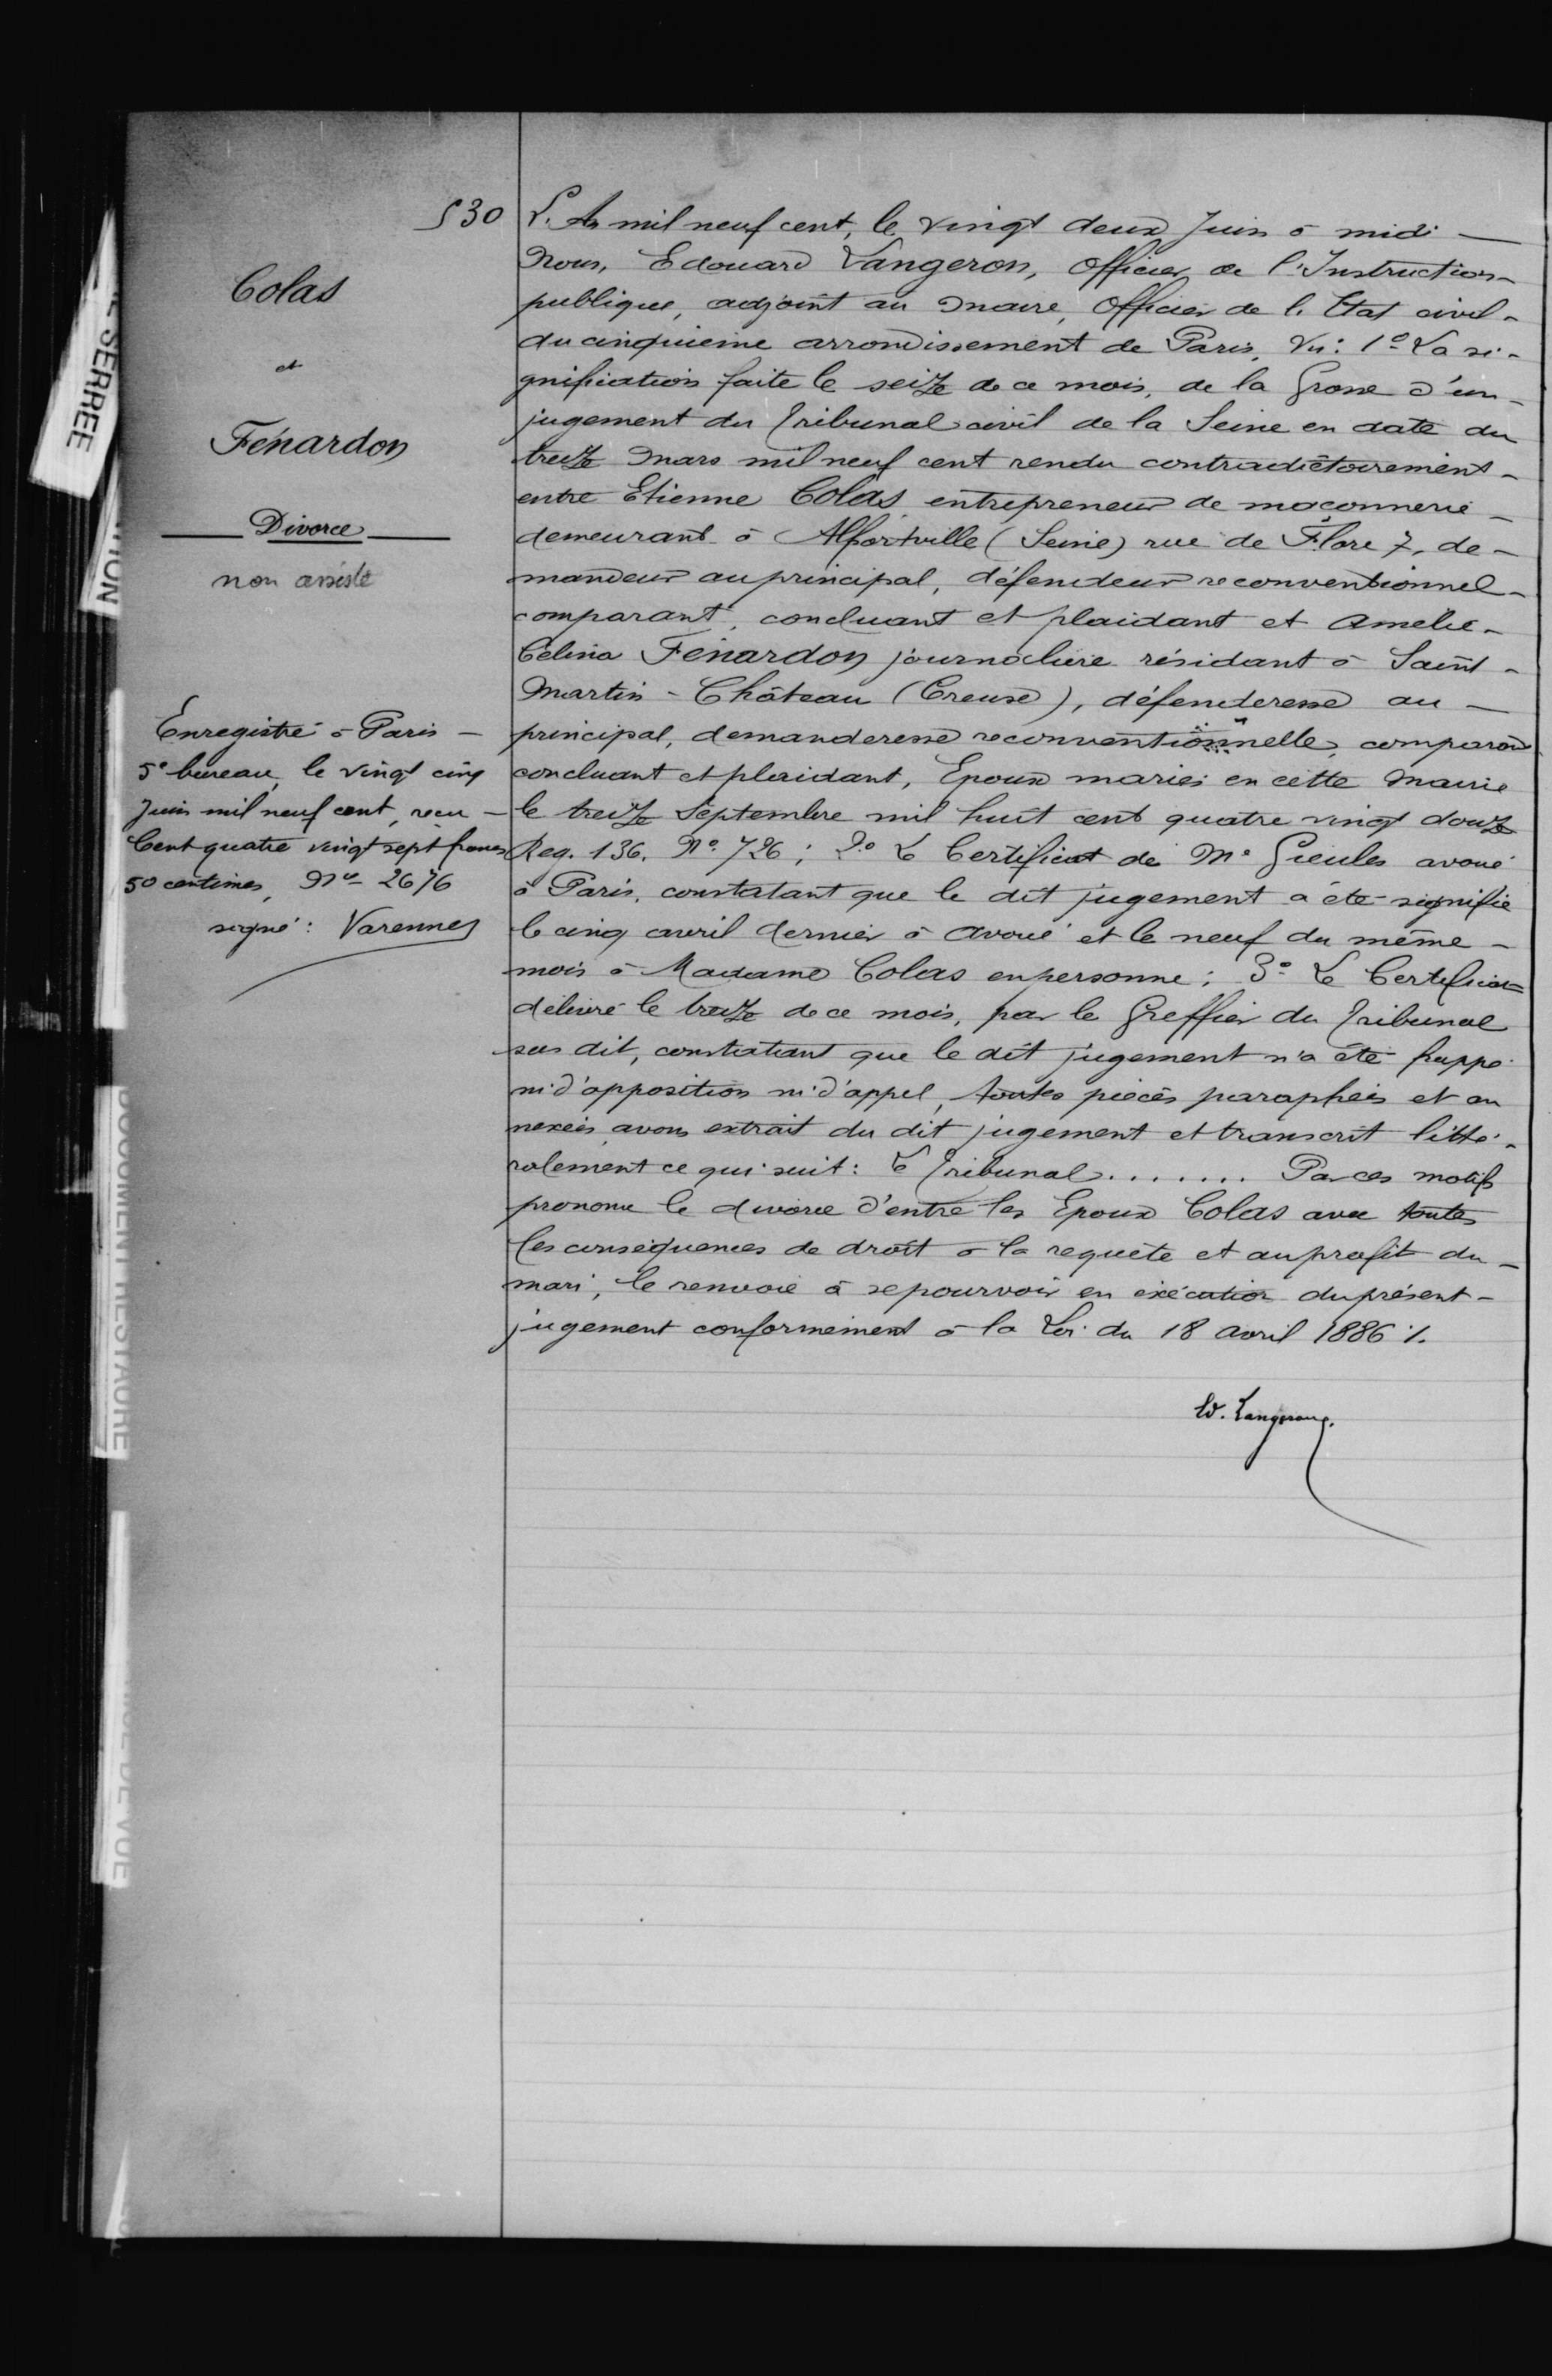530
Colas
et
Fénardon
Divorce
non assisté
L'An mil neuf cent le vingt deux Juin à midi -
Nous, Edouard Langeron, Officier de l'Instruction
publique, adjoint au maire, officier de l'Etat civil-
du cinquième arrondissement de Paris, Vu: I° La si-
gnification faite le seize de ce mois, de la Grosse d'un-
jugement du Tribunal civil de la Seine en date du
treize mars mil neuf cent rendu contradictoirement-
entre Etienne Colas entrepreneur de maçonnerie-
demeurant à Alfortville (Seine) rue de Flore 7, de-
mandeur au principal, défendeur reconventionnel-
comparant, concluant et plaidant et Amelie-
Celine Fenardon journaliere résidant à Saint-
Martin-Château (Creuse), défenderesse au-
principal, demanderesse reconventionnelle comparant
concluant et plaidant, Epoux mariés en cette mairie
le treize septembre mil huit cent quatre vingt douze
Reg. 136. N°726: 2° Le Certificat de M° Gieules avoué
à Paris, constatant que le dit jugement a été signifié
le cinq avril dernier à Avoué et le neuf du même-
mois à Madame Colas en personne: 3° Le Certificat
délivré le treize de ce mois, par le Greffier du Tribunal
sus dit, constatant que le dit jugement n'a été frappé
ni d'opposition ni d'appel, toutes pièces paraphées et an
nexées avons extrait du dit jugement et transcrit litté-
ralement ce qui suit: Le Tribunal ..... Par ces motifs
prononce le divorce d'entre les Epoux Colas aux toutes
les conséquences de droit & la requête et au profit du
mari: le renvoie à se pourvoir en exécution du présent-
jugement conformément à la Loi du 18 avril 1886 ./.
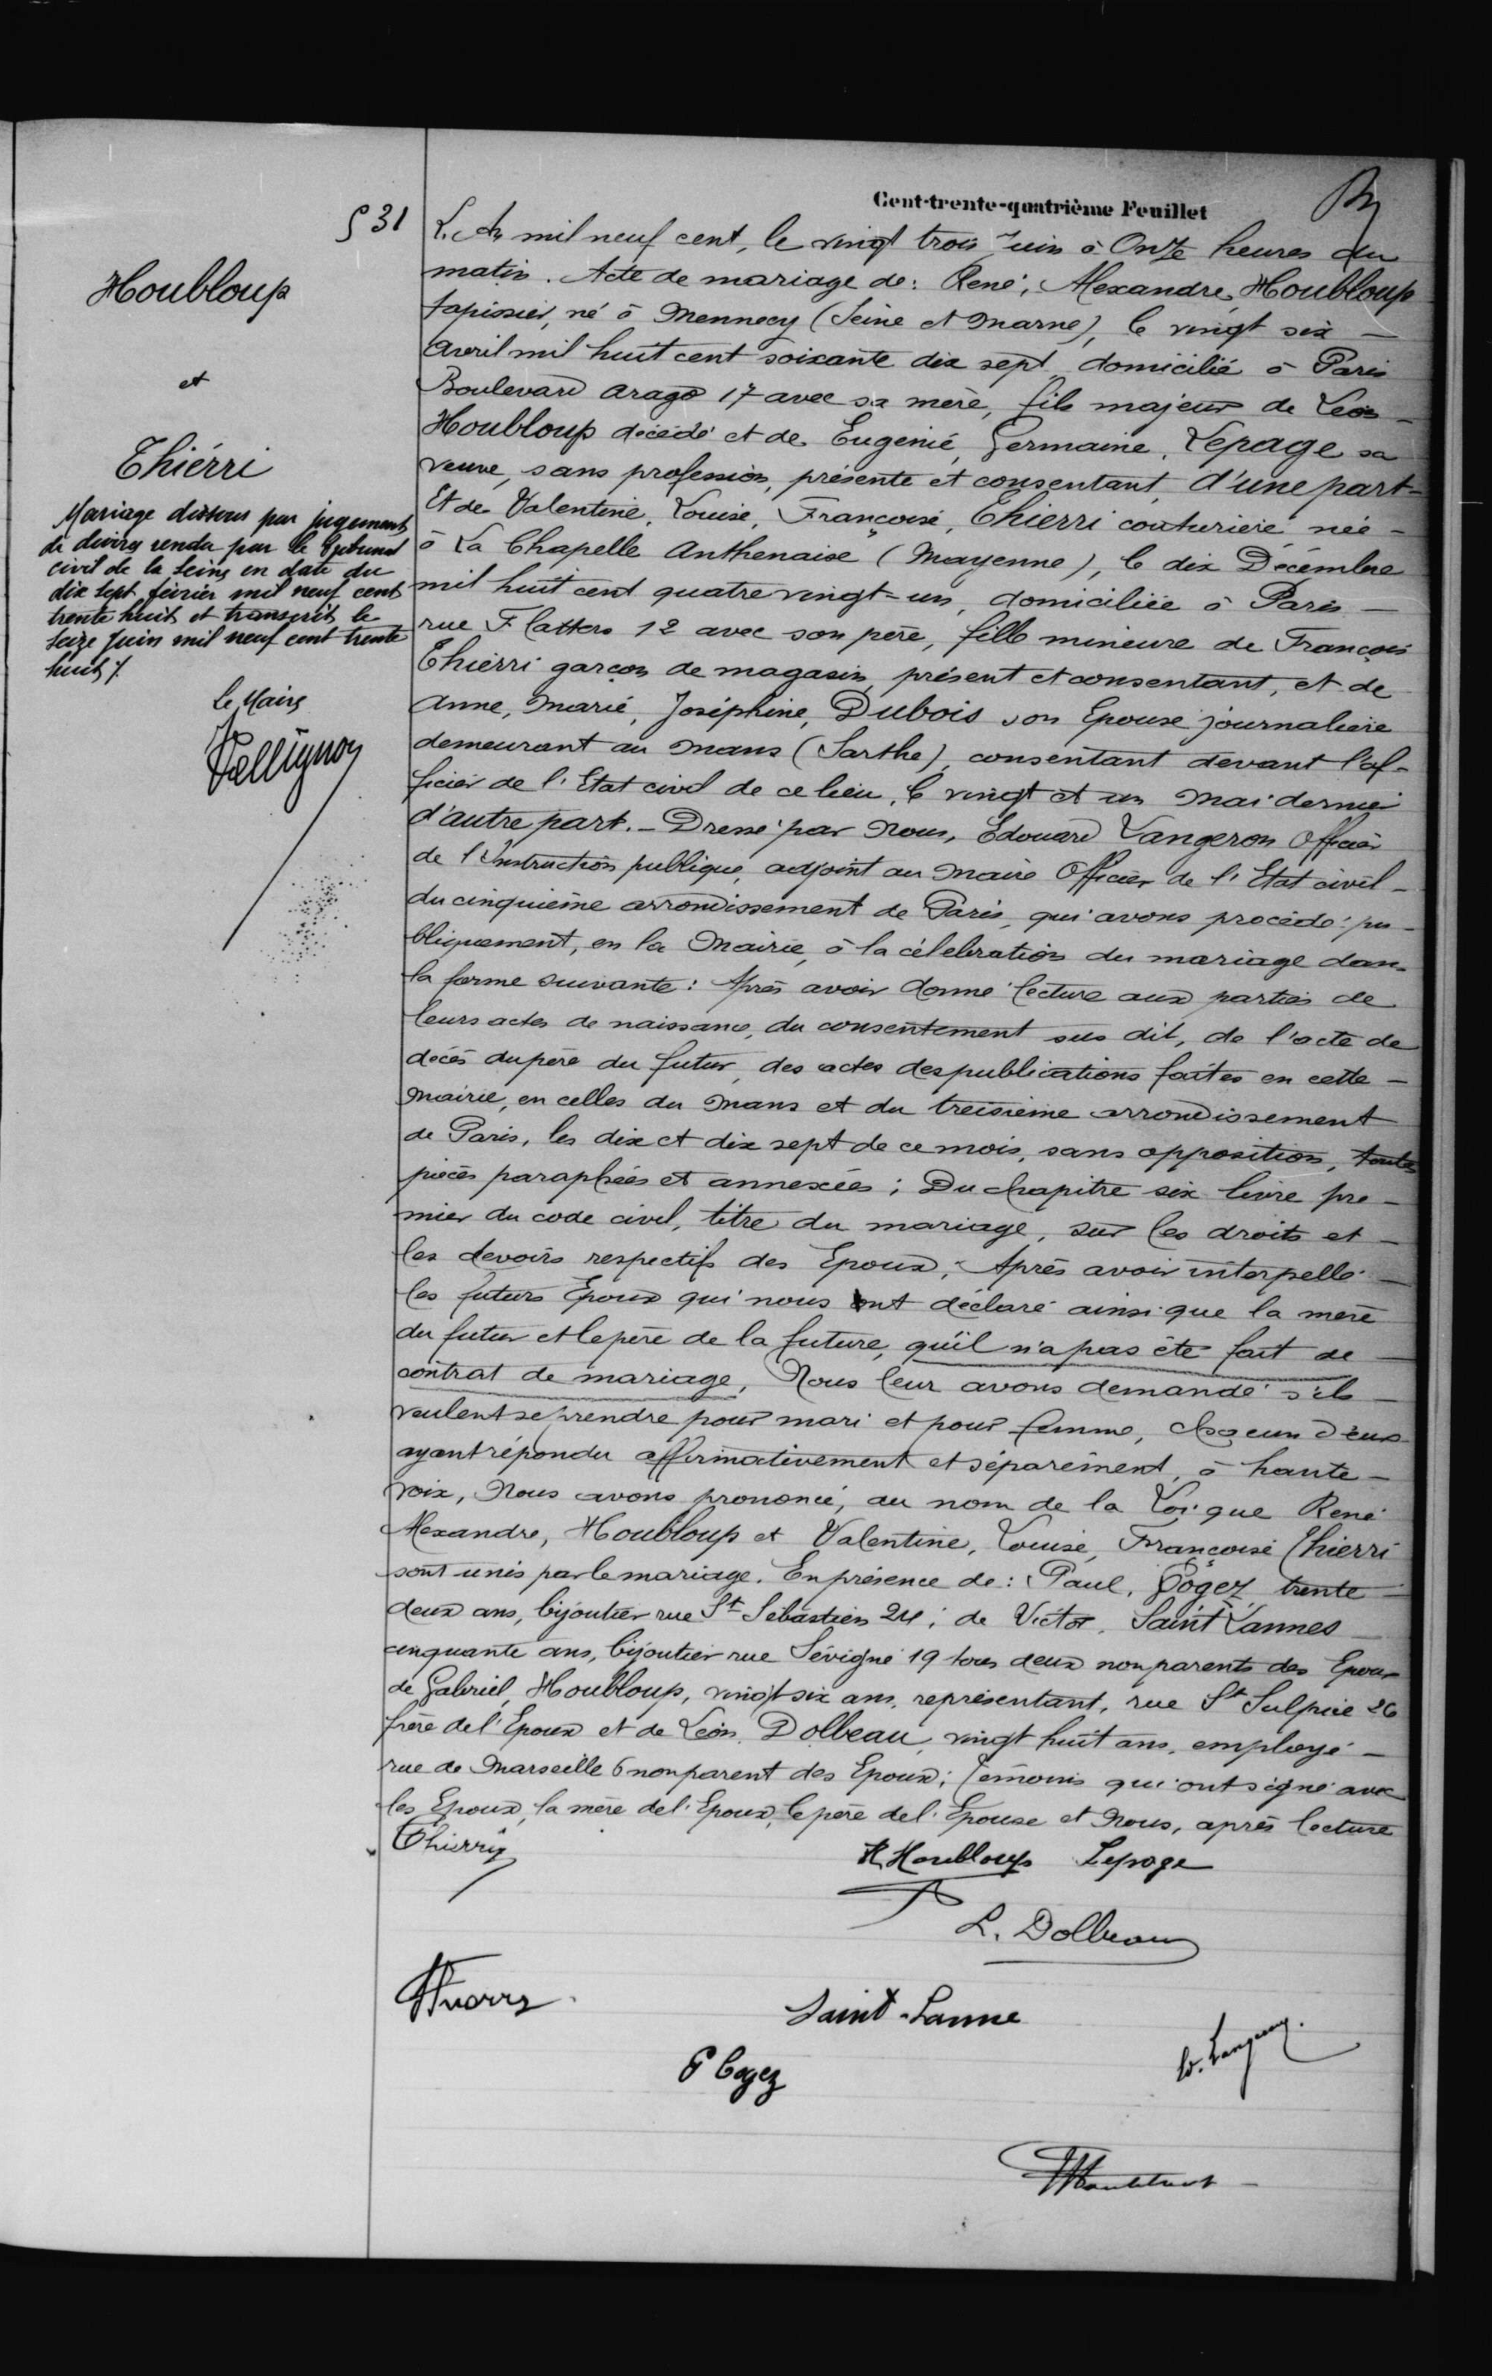531
Houbloup
et
Thiérri
L'An mil neuf cent, le vingt trois Juin à Onze heures du
matin. Acte de mariage de: René, Alexandre, Houbloup
tapissier, né à Mennecy (Seine et Marne) le vingt six-
avril mil huit cent soixante dix sept domicilié à Paris
Boulevard arago 17 avec sa mère, fils majeur de Léon
Houbloup décédé et de Eugénie Germaine Lepage sa
veuve, sans profession, présente et consentant, d'une part-
Et de Valentine, Louise, Françoise, Thierri couturière née-
à La Chapelle Anthenaise (Mayenne), le dix Décembre
mil huit cent quatre vingt-un, domiciliée à Paris-
rue Flatters 12 avec son père, fille mineure de François
Thierri garçon de magasin, présent et consentant, et de
Anne, Marie, Joséphine, Dubois son Epouse journalière
demeurant au Mans (Sarthe) consentant devant l'of-
ficier de l'Etat civil de ce lieu, le vingt et un mai dernier
d'autre part.-Dressé par Nous, Edouard Langeron Officier
de L'Instruction publique adjoint au Maire Officier de l'Etat civil-
du cinquième arrondissement de Paris, qui avons procédé pu-
bliquement, en la Mairie, à la célébration du mariage dans
la forme suivante: Après avoir donné lecture aux parties de
leurs actes de naissance, du consentement sus dit, de l'acte de
décès du père du futur, des actes des publications faites en cette-
mairie, en celles du Mans et du treisième arrondissement
de Paris, les dix et dix sept de ce mois, sans opposition, toutes
pièces paraphées et annexées: Du chapitre six livre pre-
mier du code civil, titre du mariage, sur les droits et
les devoirs respectifs des Epoux: Après avoir interpellé
les futurs Epoux qui nous ont déclaré ainsi que la mère
du futur et le père de la future qu'il n'a pas été fait de
contrat de mariage, Nous leur avons demandé s'ils-
veulent se prendre pour mari et pour femme, chacun d'eux
ayant répondu affirmativement et séparément à haute-
vois, Nous avons prononcé au nom de la Loi que René
Aleaxndre, Houbloup et Valentine, Louise, Françoise Thierri
sont unis par le mariage. En présence de: Paul, Roger, trente-
deux ans, bijoutier rue St Sébastien 24: de Victor, Saint Lannes
cinquante ans, bijoutier rue Sévigne 19 tous deux non parents des Epoux
de Gabriel Houbloup, vingt six ans, représentant, rue St Sulpice 26
frère de l'Epoux et de Léon Dolbeau vingt huit ans, employé
rue de Marseille 6 non parent des Epoux; témoins qui ont signé avec
les Epoux, la mère de l'Epoux, le père de l'Epouse et Nous, après lecture